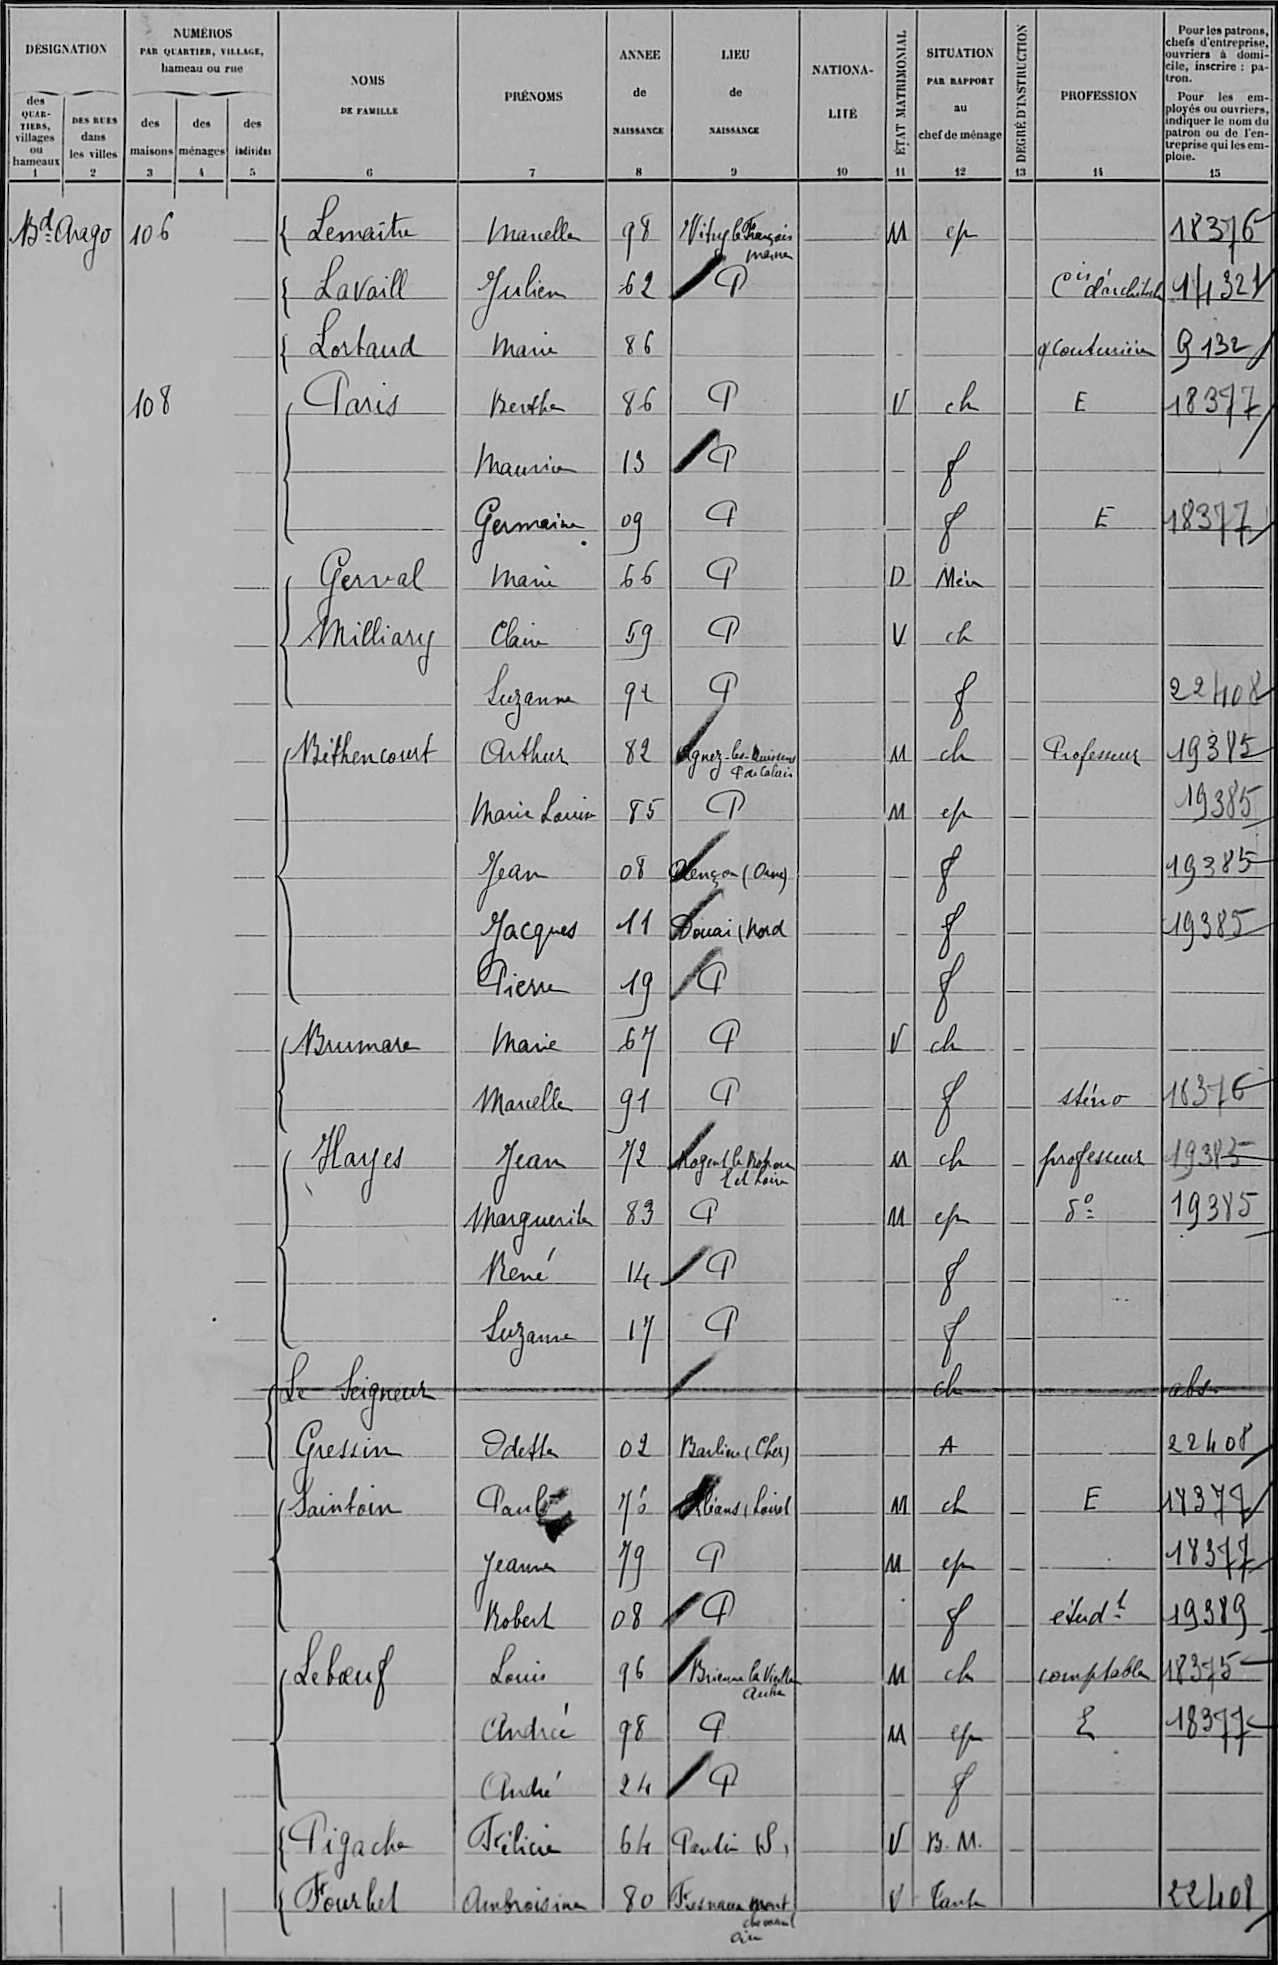Lemaître/marcelle/98/Vitry le François!Marne/¤/M/Ep/¤/¤/18376
Lavaill/Julien/62/P/¤/¤/¤/¤/C d'architecture/14321
Lortaud/Marie/86/¤/¤/¤/¤/¤/o couturiere/9132
Paris/Berthe/86/P/¤/V/ch/¤/E/18377
¤/Maurice/13/P/¤/¤/f/¤/¤/¤
¤/Germaine/09/P/¤/¤/f/¤/E/18377
Gerval/Marie/66/P/¤/D/Mère/¤/¤/¤
Milliary/Claire/59/P/¤/V/ch/¤/¤/¤
¤/Suzanne/92/P/¤/¤/f/¤/¤/22408
Béthencourt/Arthur/82/Agnez-les-Duisans!P de Calais/¤/M/ch/¤/Professeur/19385
¤/Marie Louise/85/P/¤/M/ep/¤/¤/19385
¤/Jean/08/Alençon (Orne)/¤/¤/f/¤/¤/19385
¤/Jacques/11/Douai (Nord/¤/¤/f/¤/¤/19385
¤/Pierre/19/P/¤/¤/f/¤/¤/¤
Brumare/Marie/67/P/¤/V/ch/¤/¤/¤
¤/Marcelle/91/P/¤/¤/f/¤/sténo/18376
Hayes/Jean/72/nogent le rotrou!E et Loir/¤/M/ch/¤/professeur/19385
¤/Marguerite/83/P/¤/M/ep/¤/d°/19385
¤/René/14/P/¤/¤/f/¤/¤/¤
¤/Suzanne/17/P/¤/¤/f/¤/¤/¤
Le Seigneur/¤/¤/¤/¤/¤/ch/¤/¤/abs
Gressin/Odette/02/Barlieu (Cher)/¤/¤/A/¤/¤/22408
Saintoin/Paul/76/Orléans (Loiret)/¤/M/ch/¤/E/18377
¤/Jeanne/79/P/¤/M/ep/¤/¤/18377
¤/Robert/08/P/¤/¤/f/¤/étudt/19389
Leboeuf/Louis/96/Brienne la vieille!Aube/¤/M/ch/¤/comptable/18375
¤/Andrée/98/P/¤/M/ep/¤/E/18377
¤/André/24/P/¤/¤/f/¤/¤/¤
Pigache/Félicie/64/Pantin (S)/¤/V/B M /¤/¤/¤
Fourhet/Ambroisine/80/Fresnaux Mon !chevreuil!Ain/¤/V/tante/¤/¤/22408
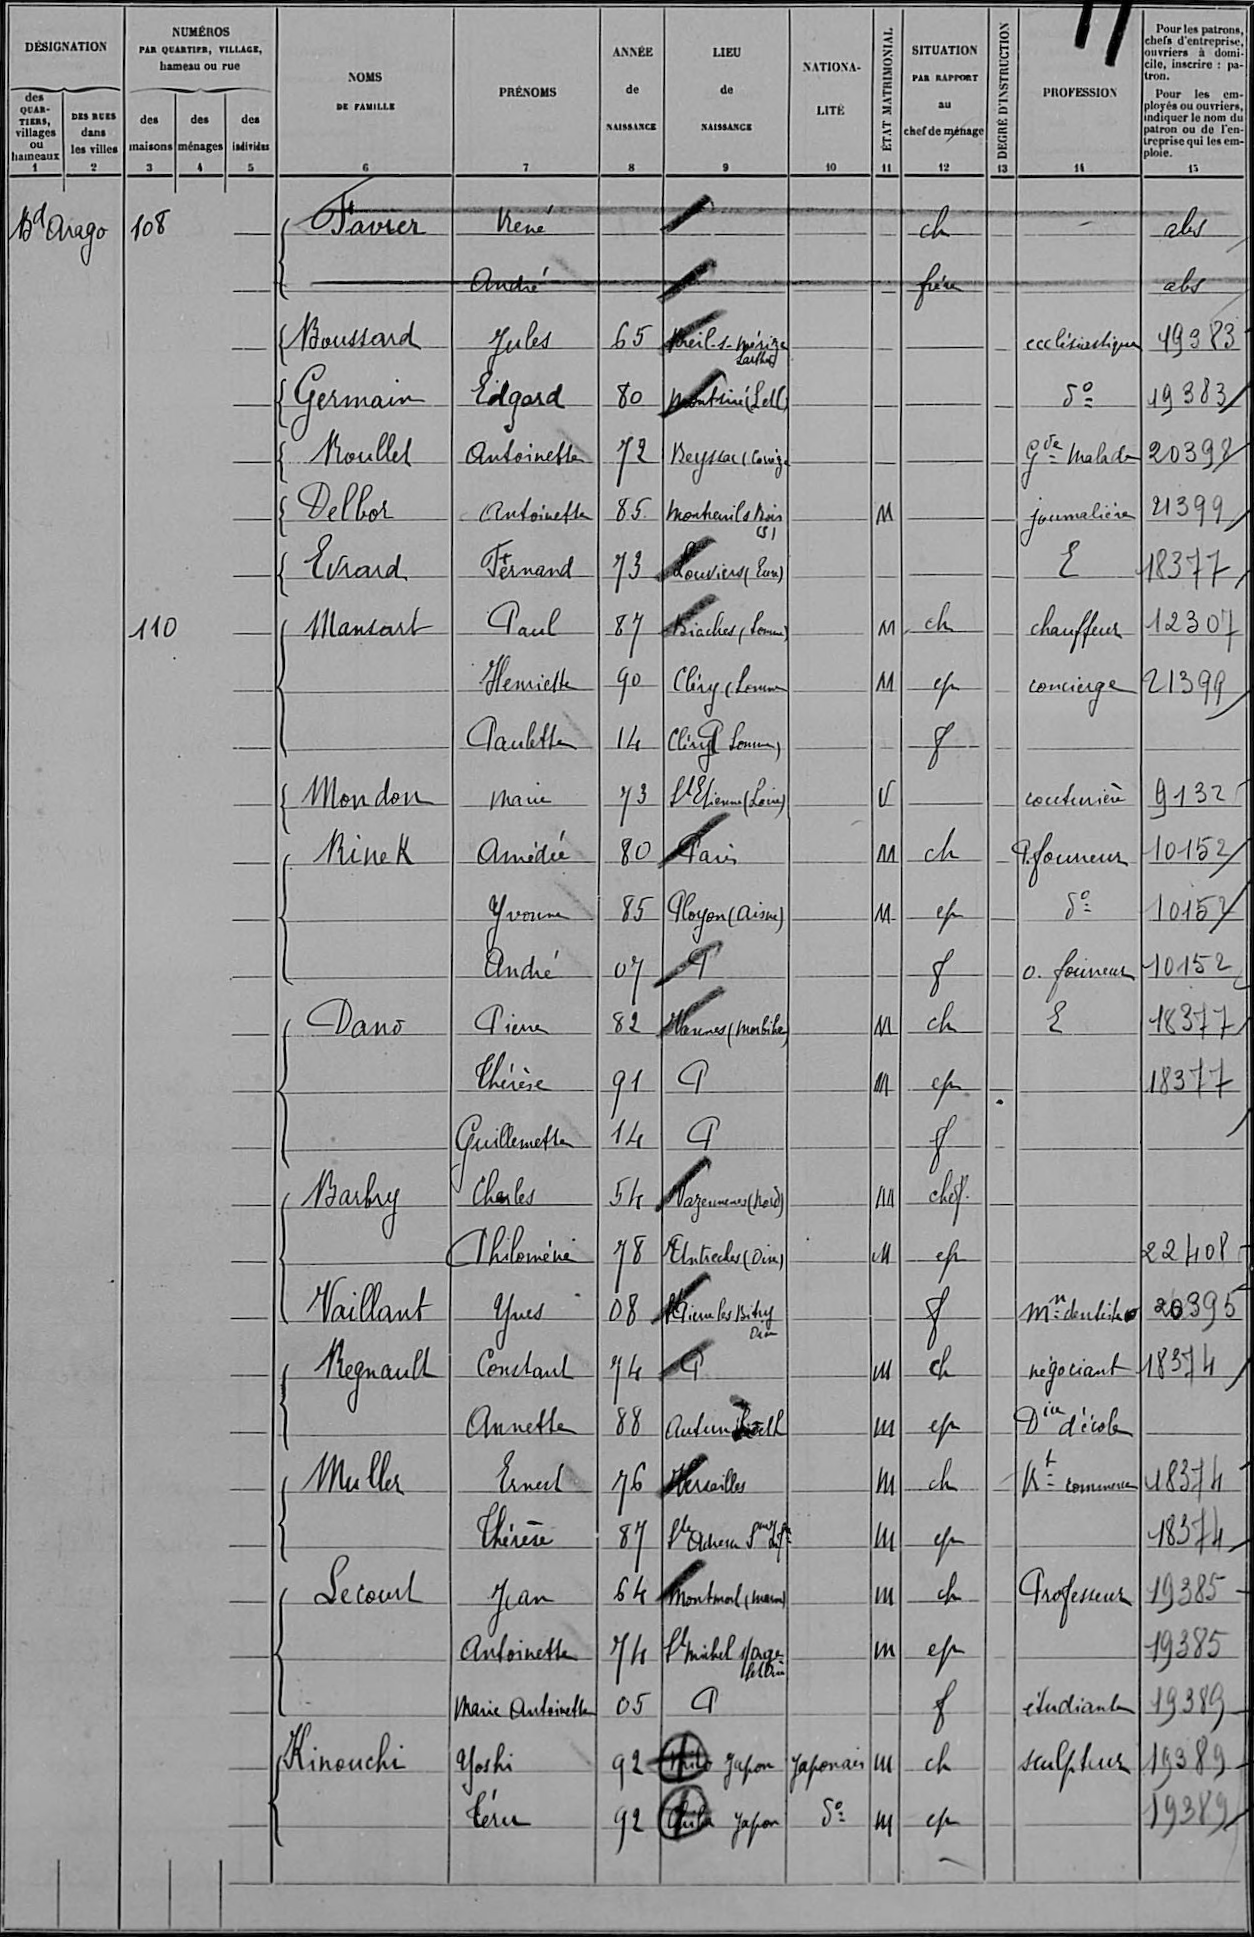Favier/René/¤/¤/¤/¤/ch/¤/¤/abs
¤/André/¤/¤/¤/¤/frère/¤/¤/abs
Boussard/Jules/65/Breil-sur-Mérize! Sarthe/¤/¤/¤/¤/ecclesiastique/19383
Germain/Edgard/80/Montrieux (L et C)/¤/¤/¤/¤/d°/19383
Rouller/Antoinette/72/Beyssac (Corrèze/¤/¤/¤/¤/Gde malade/20398
Delbor/Antoinette/85/Montreuil s Bois!(S)/¤/M/¤/¤/journalière/21399
Evrard/Fernand/73/Louviers (Eure)/¤/¤/¤/¤/E/18377
Mansart/Paul/87/Biaches (Somme)/¤/M/ch/¤/chauffeur/12307
¤/Henriette/90/Cléry (Somme/¤/M/ep/¤/concierge/21399
¤/Paulette/14/Cléry (Somme)/¤/¤/f/¤/¤/¤
Mondon/Marie/73/St Etienne (Loire)/¤/V/¤/¤/couturière/9132
Rinek/Amedée/80/Paris/¤/M/ch/¤/P fourreur/10152
¤/Yvonne/85/Noyon (Aisne)/¤/M/ep/¤/d°/10152
¤/André/07/P/¤/¤/f/¤/o fourreur/10152
Dano/Pierre/82/Vannes (morbihan)/¤/M/ch/¤/E/18377
¤/Thérèse/91/P/¤/M/ep/¤/¤/18377
¤/Guillemette/14/P/¤/¤/f/¤/¤/¤
Barbry/Charles/54/Wazemmes (Nord)/¤/M/chef/¤/¤/¤
¤/Philomène/78/Autreches (Oise)/¤/M/ep/¤/¤/22408
Vaillant/Yves/08/St Pierre les Bitry!Oise/¤/¤/f/¤/Mn dentiste/20395
Regnault/Constant/74/P/¤/M/ch/¤/négociant/18374
¤/Annette/88/Autun S et L/¤/M/ep/¤/Dice d'école/¤
Muller/Ernest/76/Versailles/¤/M/ch/¤/Rt commerce/18374
¤/Thérèse/87/Ste Adresse Sne Inf/¤/M/ep/¤/¤/18374
Lecourt/Jean/64/Montmort (Marne)/¤/m/ch/¤/Professeur/19385
¤/antoinette/74/St Michel s-orge!S et Oise/¤/m/ep/¤/¤/19385
¤/Marie Antoinette/05/P/¤/¤/f/¤/étudiante/19389
Kinouchi/Yoshi/92/Khito Japon/Japonais/m/ch/¤/sculpteur/19389
¤/Céru/92/Chiba Japon/d°/m/ep/¤/¤/19389